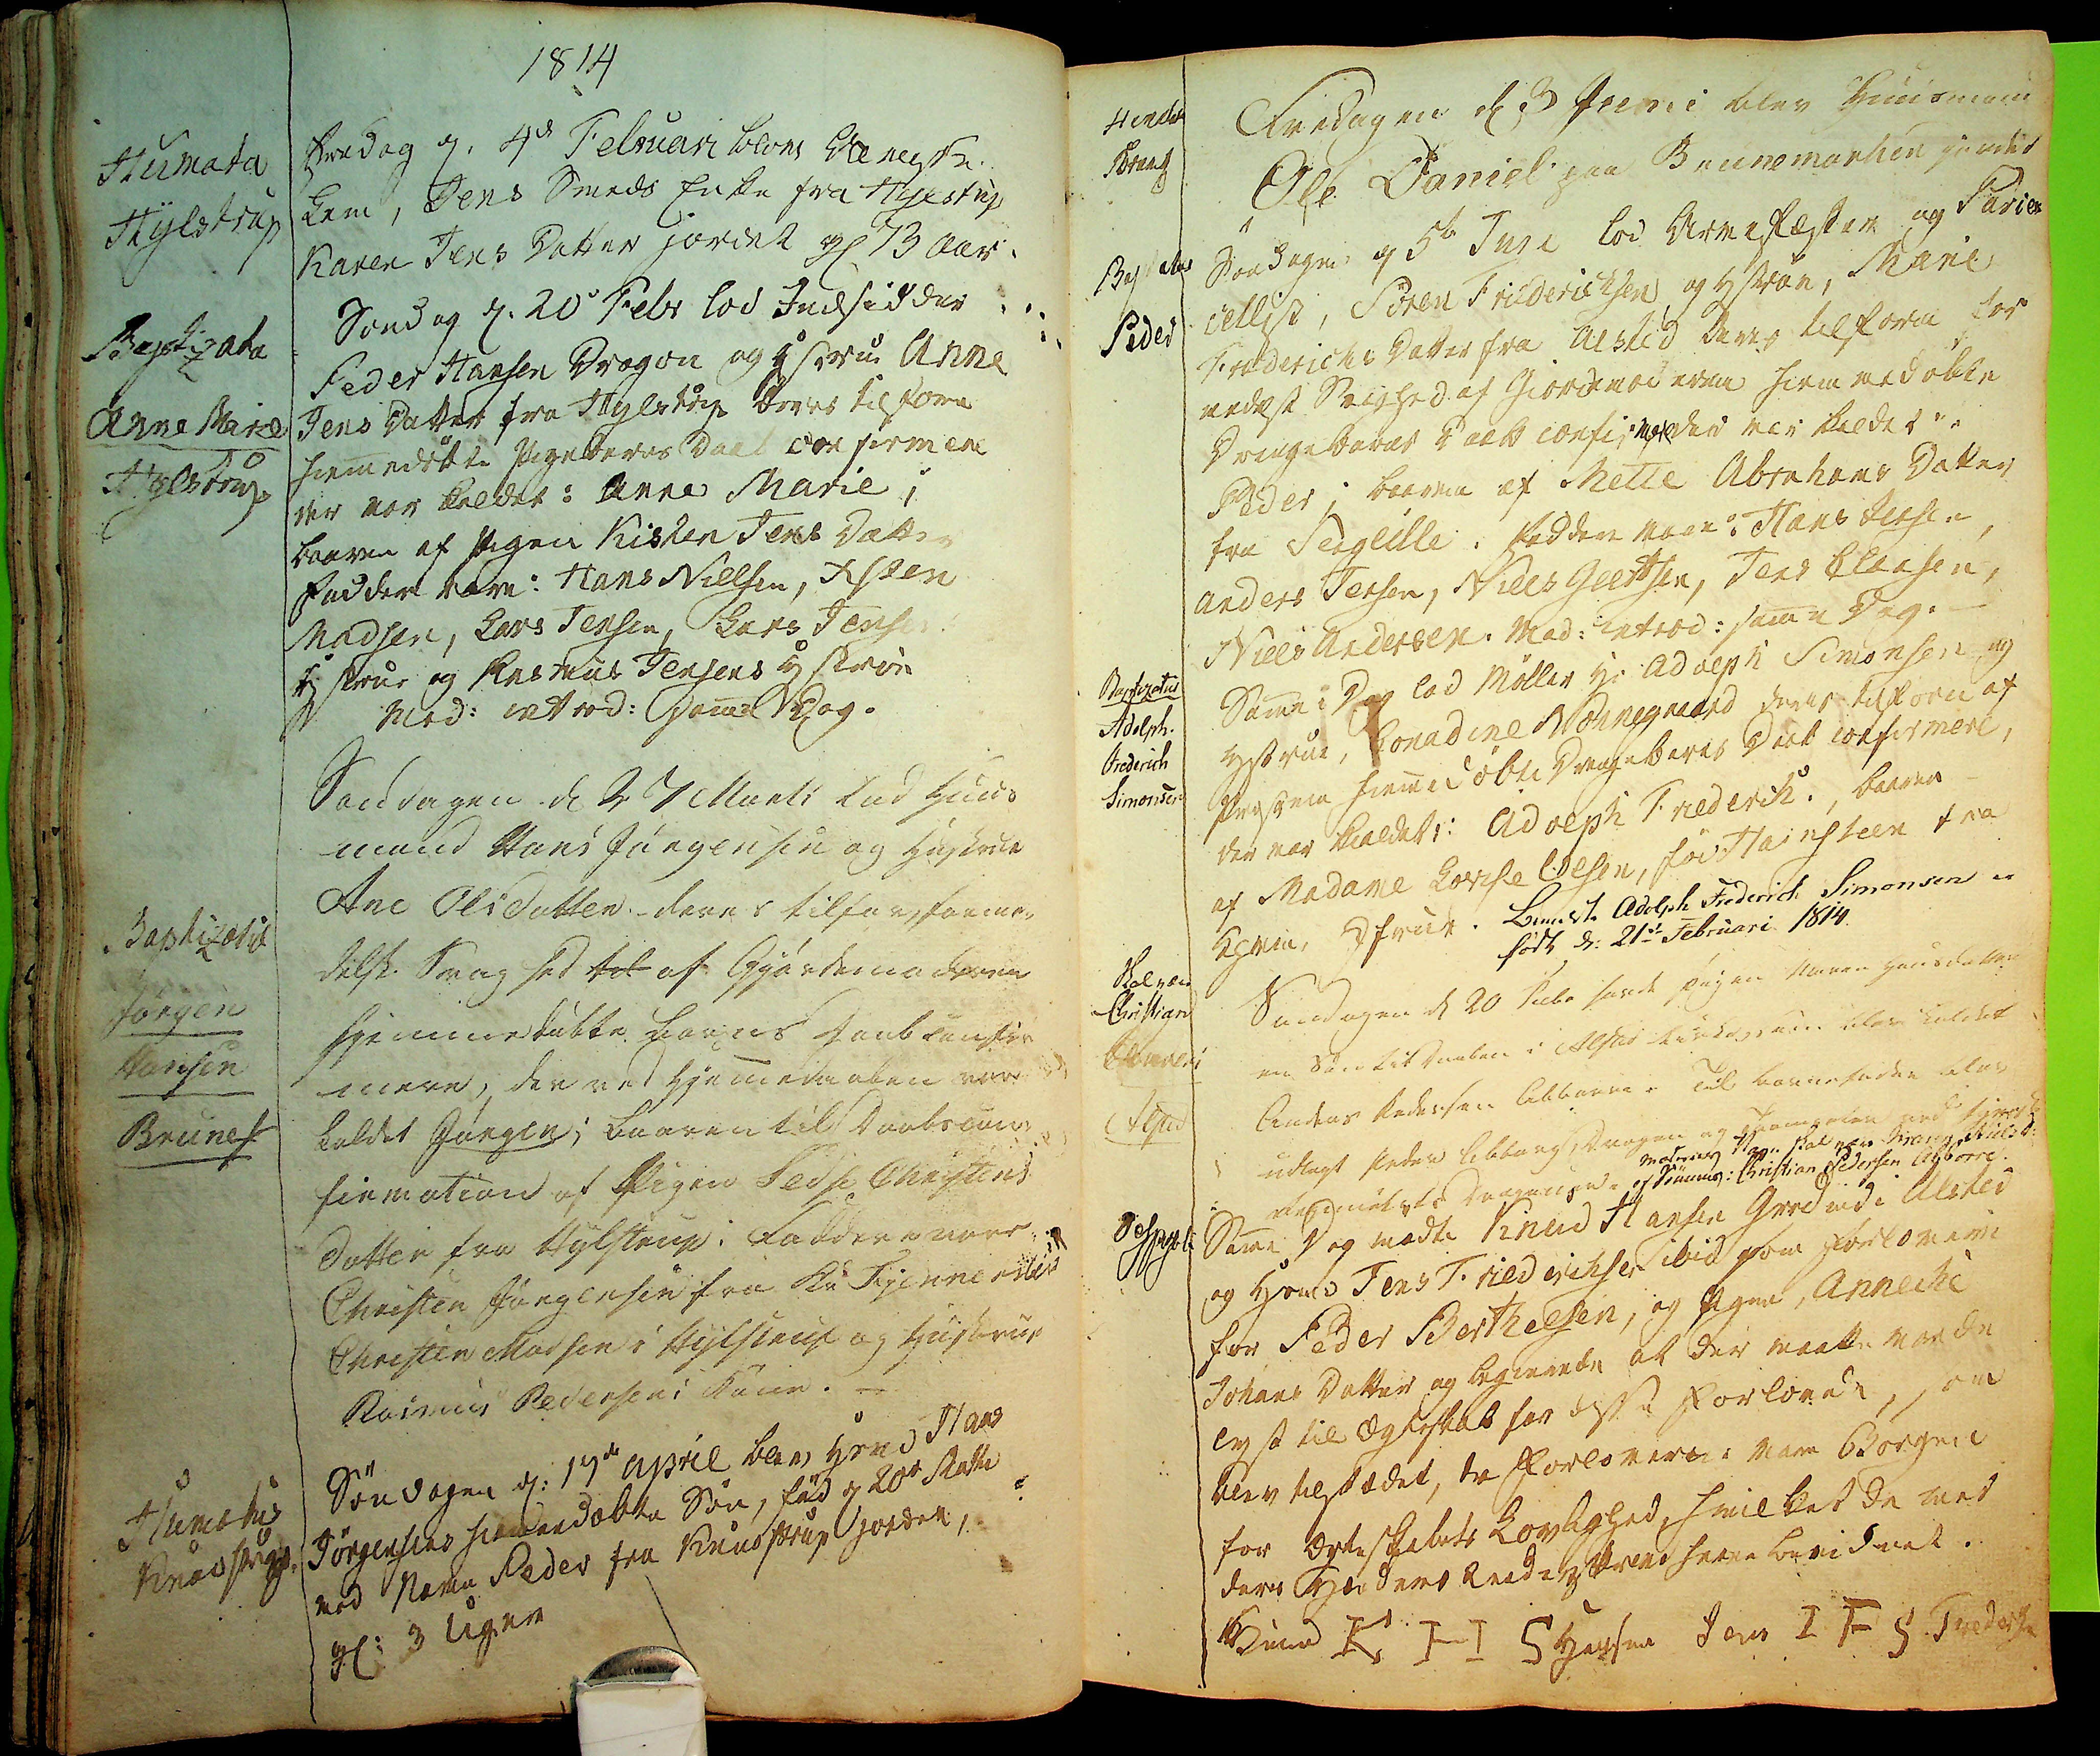Humata
Hylstrup
Baptizata
Anne Marie
Hylstrup
Baptizatus
Jørgen
Hansen
Brune##
Humatus
Knudstrup.
1814
Fredag d 4de Februari blev Almisse
Lem, Jens Smeds Enke fra Hylstrup
Karen Jens Datter jordet gl 73 Aar.
Søndag d: 20de Febr lod Indsidder
Peder Hansen Draogum og Hstrue Anne
Jens Datter fra Hylstrup deres tilforn
hiemmedøbt Pigebarns Daab confirmere
der var kaldet: Anne Marie;
baaren af Pigen Kirsten Jens Datter
Faddere vare: Hans Nielssen, Xsten
Madsen, Lars Jensen, Lars Jensens
Hstrue og Rasmus Jensens Hstrue
Mod: introd: samme dag.
Søndagen d 27 Marts lod Huus
mand Hans Jørgensen og Hustrue
Anne Olsdatter deres tilforn forme¬
dels Svaghed til af Gjordemoderen
hjemmedøbte Barns Daab confir
amere, der ved Hiemedaaben var
kaldet Jørgen; baaren til Daabscon
firmation af Pigen Sedse Christens
Datter fra Hylstrup. Faddere vare:
Christen Jørgensen fra Kr Fjenneslev
Christen Madsen i Hylstrup og Hustrue
Rasmus Pedersens Kone
Søndagen d: 17de April blev Hsmd Hans
Jørgensens hiemmedøbte Søn, fød d 20de Marts
ved Navn Peder fra Knudstrup jordet,
gl: 3 Uger
Humatus
Brunem##
Baptizatus
Peder
Baptizatus
Adolph.
Friderich
Simonsen
##ol##
Christian
##
Alsted
Desponsati
Fredagen d: 3 Juni blev Huusmand
Else Daniel paa Brunemarken jordet
Søndagen d 5te Juni lod Arvefæster og Parce
cellist, Søren Friderichsens Hstrue, Marie
Friderichs Datter fra Alsted deres tilforn for
medelst Svaghed af Giordemoderen hiemmedøbte
Drengebarns Daab confirmere der var kaldet 
Peder, baaren af Mette Abrahams Datter
fra Slaglille. Faddere vare: Hans Jensen,
Anders Jensen, Niels Geertsen, Jens Clausen,
Niels Andersen. Mod: introd: samme Dag.
Samme Dag lod Møller H: Adolph Simonsen og
Hstrue, Conadine Nonnegaard deres tilforen af
Præsten hiemmedøbte Drengebarns Daab confirmere,
der var kaldet: Adolph Frederich., baaren
naf Madame Lovise Olsen, fød Ha##steen fra
J## Jfrue. Bemeldte Adolph Frederuch Simonsen er
født d: 21de Februari 1814.
Søndagen d 20 Febr havde Pigen Maren Hansdatter
en Søn til Daaben i Alsted Kirke som blev kaldet
Anders Pedersen Abborre. Til barnefader blev
udlagt Peder Abborg, Dragon og Trompeter## ved fjerde##
Regimentets Dragoner##. 
Moderens Navn skal være Maren Niels D:
og Sønnens: Christian Pedersen Abborre
Samme Dag mødte Knud Hansen Grdmd i Alsted
og Hsmd Jens Friederichsen ibid som Forlovere
for Peder Berthelsen, og Pigen Anneche
Johans Datter og begierede at der maatte vorde
oglyst til Ægteskab for disse Forlovede, som
blev tilstædet, da Forloverne vare Borgen
for Ægteskabets Lovlighed, hvilket de med
deres Hænders Underskrivt have bevidnet.
Knud K H S Hansen Jens I F S Friedrichsen
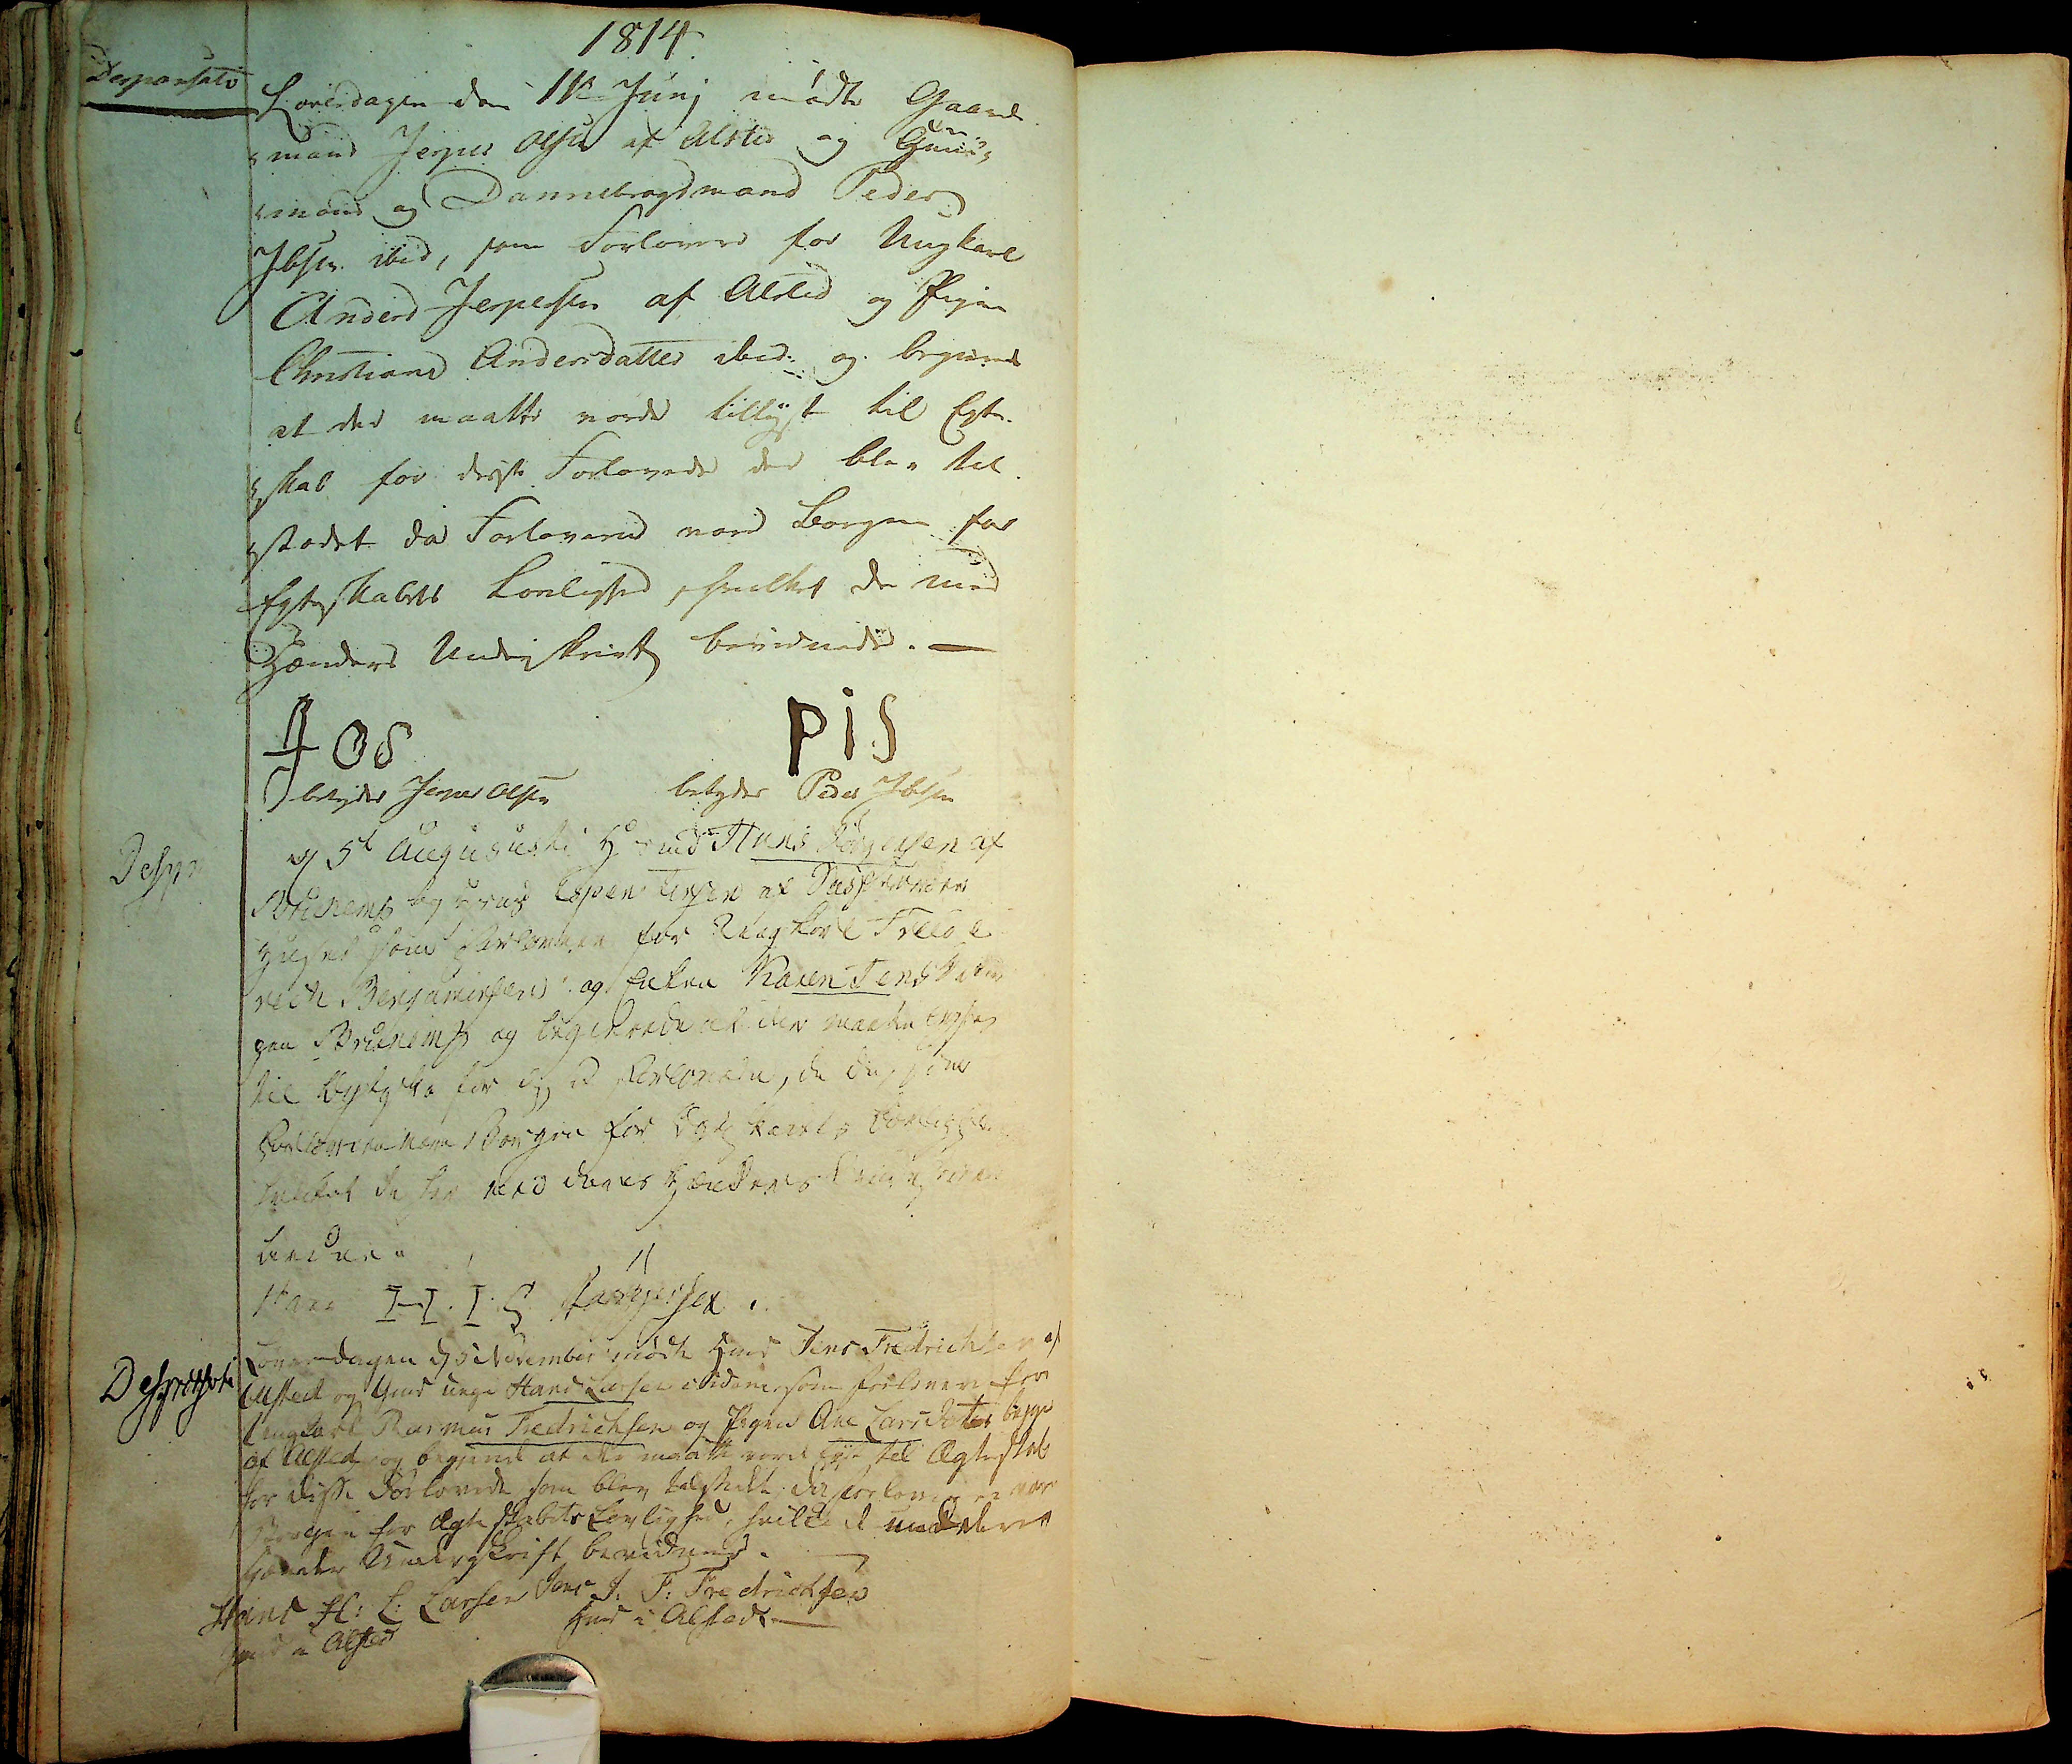Desponsati
Des##
Desponsati
1814.
Løverdagen den 11te Junj mødte Gaard
mand Jesper Olsøn af Alsted og Huus¬
mand og Dannebrogsmand Peder
Ibsen ibid, som Forlovere for Ungkarl
Anders Jespersen af Alsted og Pigen
Christiane Andersdatter ibid: og begierede
at der maatte vorde tillyst til Egte¬
skab fød disse Forlovede det blev til
stædet da Forlovere vorde Borgen for
Egteskabet Lovlighed, hvilket de med
Hænders Underskrivt bevidnede.
J O S
betyder Jens Olsen
P I S
betyder Peder Ibsen
d 5te Augusti Hsmd Hans Jørgensen af
Brunem## og Hsmd Espen Jensen af Sa##
Huset, som Forlovere for Ungkarl Frede
rich Benjaminsen og Enke Karen Jens Datter
paa Brunem## og begierede at der maatelyses
til Ægteskab for disse Forlovede, da de som
Forlevere vare Borgen for Ægteskabets Lovlighed
hvilket de her med deres Hænders Underskrivt
bedne.
Hans H I S Jørgensen
Løverdagen d 5 November mødte Hmd  Jens Fredrichsen af
Alsted og Gmd unge Hans Larsen ibidem, som forlovere for
Ungkarl Rasmus Fredrichsen og Pigen Ane Lars Datter begge
af EAlsted og begjerede at der maatte vorde lyst til Ægteskab
for disse Forlovede, som blev tilstædet, da Forloverne var
TBorgen for Ægteskabets Lovlighed, hvilketde med deres
Hænders Underskrift bevidner.
Hans H: L: Larsen  Jens J: F: Frederichsen
Gmd i Alsted
Hmd i Alsted